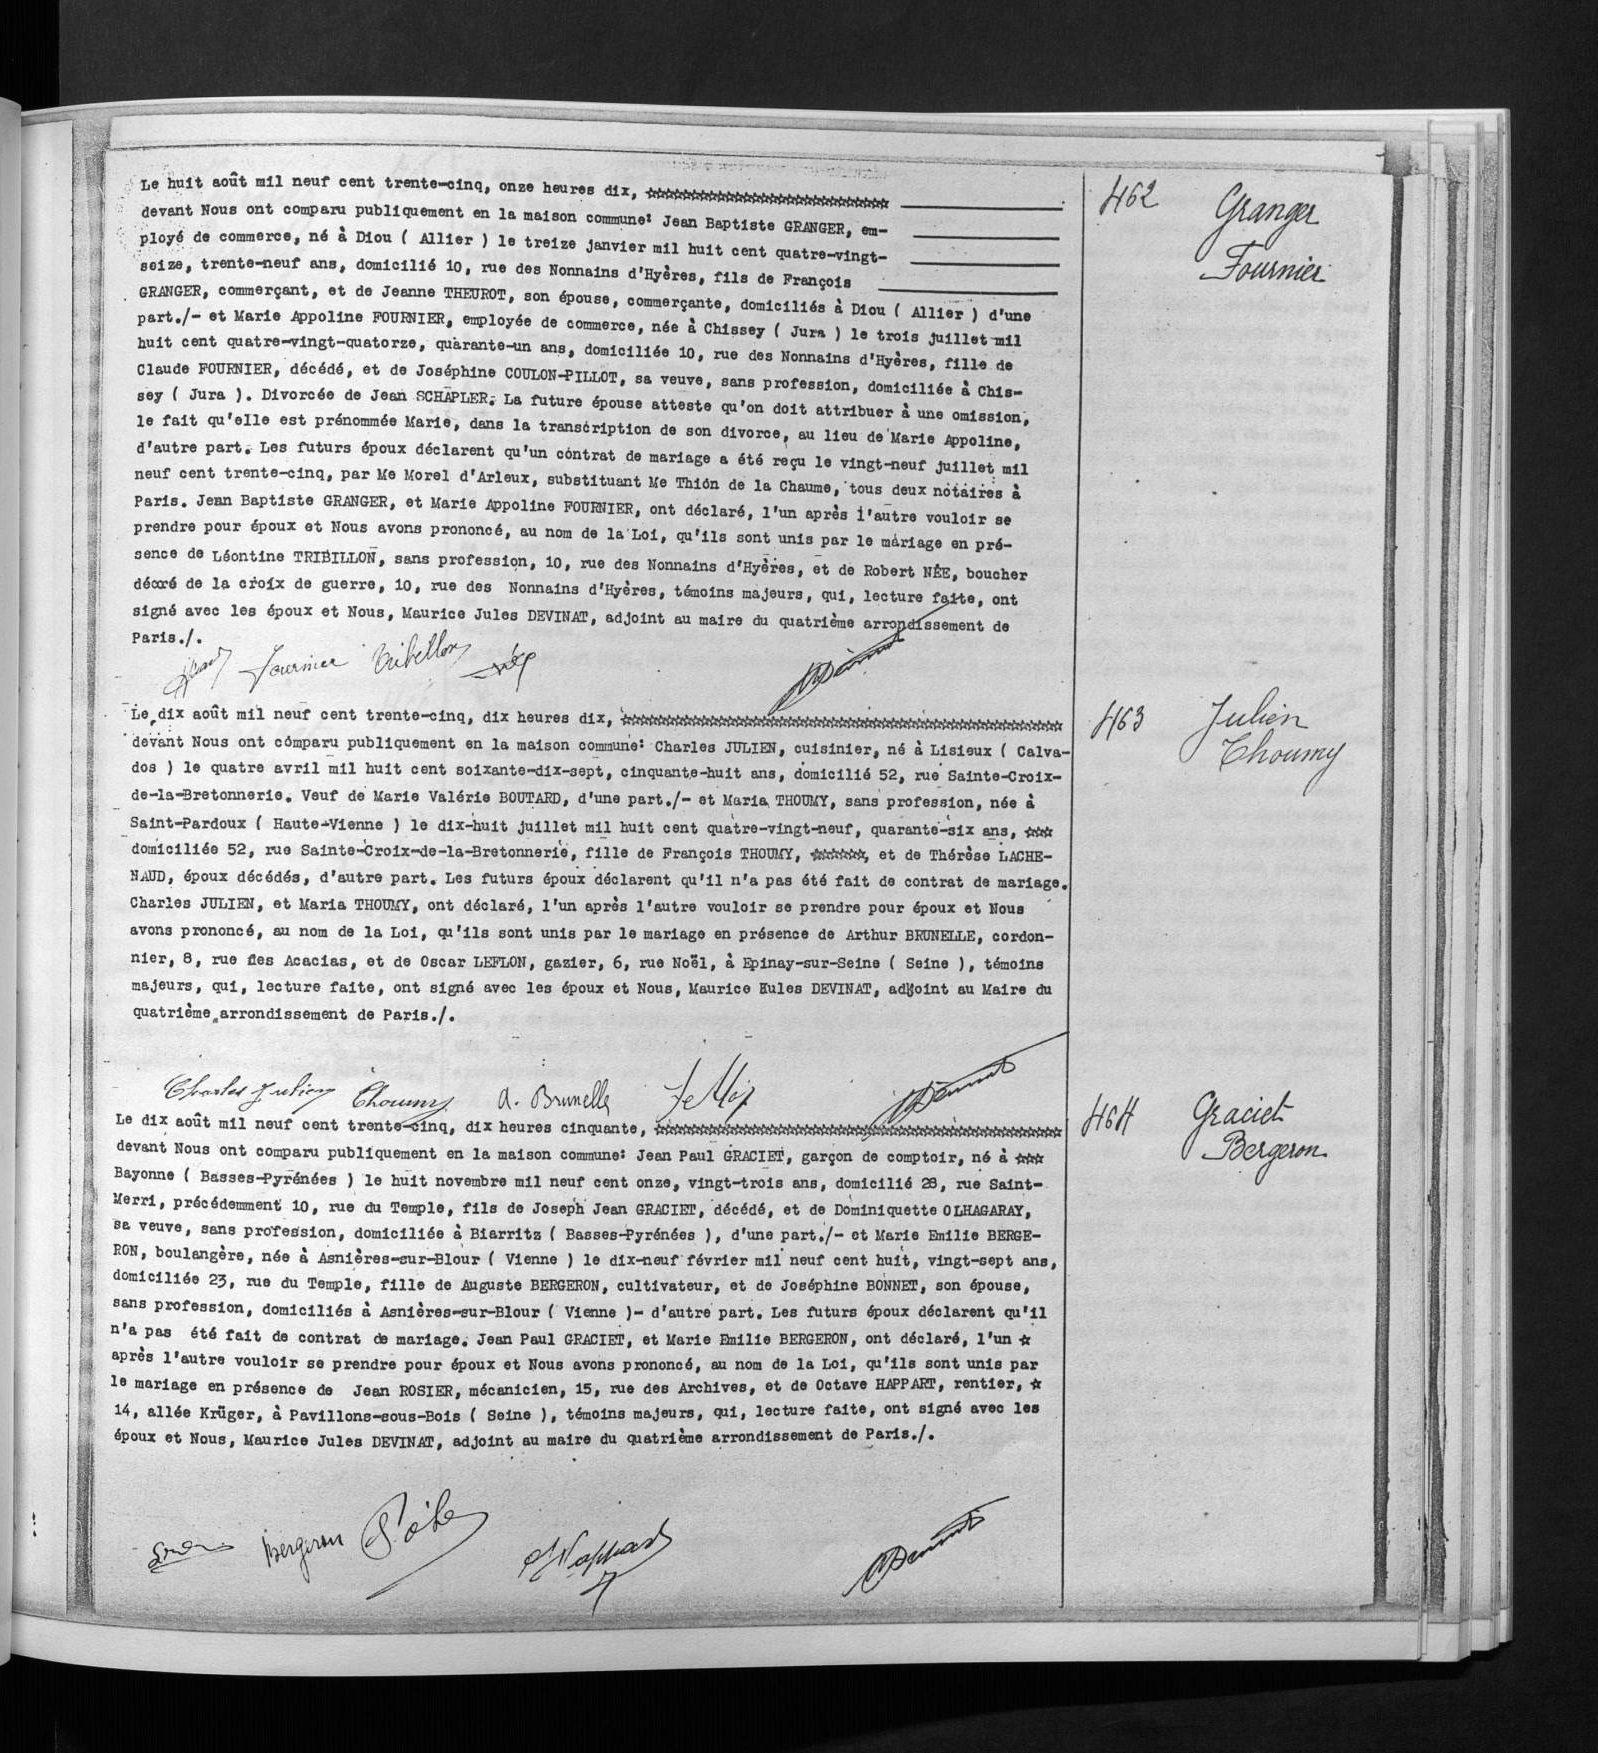Le huit août mil neuf cent trente-cinq, onze heures dix, ****-
devant Nous ont comparu publiquement en la maison commune: Jean Baptiste GRANGER, em -
mployé de commerce, né à Diou (Allier) le treize janvier mil huit cent quatre-vingt-
seize, trente-neuf ans, domicilié 10, rue des Nonnais d'Hyères, fils de François -
GRANGER, commerçant, et de Jeanne THEUROT, son épouse, commerçante, domiciliés à Diou (Allier) d'une
part ./-et Marie Appoline FOURNIER, employée de commerce, née à Chissey (Jura) le trois juillet mil
huit cent quatre-vingt-quatorze, quarante-un ans, domiciliée 10, rue des Nonnais d'Hyères, fille de
Claude FOURNIER, décédé, et de Joséphine COULON-PILLOT, sa veuve, sans profession, domiciliée à Chis-
sey (Jura). Divorcée de Jean SCHAPLER. La future épouse atteste qu'on doit attribuer à une amission,
le fait qu'elle est prénommée Marie, dans la transcription de son divorce, au lieu de Marie Appoline,
d'autre part. Les futurs époux déclarent qu'un contrat de mariage a été reçu le vingt-neuf juillet mil
neuf cent trente-cinq, par Me Morel d'Arleux, substituant Me Thion de la Chaume, tous deux notaires à
Paris. Jean Baptiste GRANGER, et Marie Appoline FOURNIER, ont déclaré, l'un après l'autre vouloir se
prendre pour époux et Nous avons prononcé, au nom de la Loi, qu'ils sont unis par le mariage en pré-
sence de Léontine TRIBILLON, sans profession, 10, rue des Nonnains d'Hyères, et de Robert NEE, boucher
décoré de la croix de guerre, 10, rue des Nonnains d'Hyères, témoins majeurs, qui, lecture faite, ont
signé avec les époux et Nous, Maurice Jules DEVINAT, adjoint au maire du quatrième arrondissement de
Paris ./.
Le dix août mil neuf cent trente-cinq, dix heures dix, ****
devant Nous ont comparu publiquement en la maison commune: Charles JULIEN, cuisinier, né à Lisieux (Calva-
dos) le quatre avril mil huit cent soixante-dix-sept, cinquante-huit ans, domicilié 52, rue Sainte-Croix-
de-la-Bretonnerie. Veuf de Marie Valérie BOUTARD, d'une part ./-et Maria THOUMY, sans profession, née à
Saint-Pardoux (Haute-Vienne) le dix-huit juillet mil huit cent quatre-vingt-neuf, quarante-six ans, ***
domiciliée 52, rue Sainte-Croix-de-la-Bretonnerie, fille de François THOUMY, ****, et de Thérèse LACHE-
NAUD, époux décédés, d'autre part. Les futurs époux déclarent qu'il n'a pas été fait de contrat de mariage.
Charles JULIEN, et Maria THOUMY, ont déclaré, l'un après l'autre vouloir se prendre pour époux et Nous
avons prononcé, au nom de la Loi, qu'il sont unis par le mariage en présence de Arthur BRUNELLE, cordon-
nier, 8, rue des Acacias, et de Oscar LEFLON, gazier, 6, rue Noël, à Epinay-sur-Seine (Seine), témoins
majeurs, qui, lecture faite, ont signé avec les époux et Nous, Maurice Hules DEVINAT, adjoint au Maire du
quatrième arrondissement de Paris ./.
Le dix août mil neuf cent trente-cinq, dix heures cinquante, ****
devant Nous ont comparu publiquement en la maison commune: Jean Paul GRACIET, garçon de comptoir, né à **
Bayonne (Basses-Pyrénées) le huit novembre mil neuf cent onze, vingt-trois ans, domicilié 28, rue Saint-
Merri, précédemment 10, rue du Temple, fils de Joseph Jean GRACIET, décédé, et de Dominiquette OLHARGARAY,
sa veuve, sans profession, domiciliée à Biarritz (Basses-Pyrénées), d'une part ./-et Marie Emilie BERGE-
RON, boulangère, née à Asnière-sur-Blour (Vienne) le dix-neuf février mil neuf cent huit, vingt-sept ans,
domiciliée 23, rue du Temple, fille de Auguste BERGERON, cultivateur, et de Joséphine BONNET, son épouse,
sans profession, domiciliés à Asnière-sur-Blour (Vienne)-d'autre part. Les futurs époux déclarent qu'il
n'a pas été fait de contrat de mariage. Jean Paul GRACIET, et Marie Emilie BERGERON, ont déclaré, l'un *
après l'autre vouloir se prendre pour époux et Nous avons prononcé, au nom de la Loi, qu'ils sont unis par
le mariage en présence de Jean ROSIER, mécanicien, 15, rue des Archives, et de Octave HAPPART, rentier, *
14, allée Krüger, à Pavillons-sous-Bois (Seine), témoins majeurs, qui, lecture faite, ont signé avec les
époux et Nous, Maurice Jules DEVINAT, adjoint au maire du quatrième arrondissement de Paris ./.
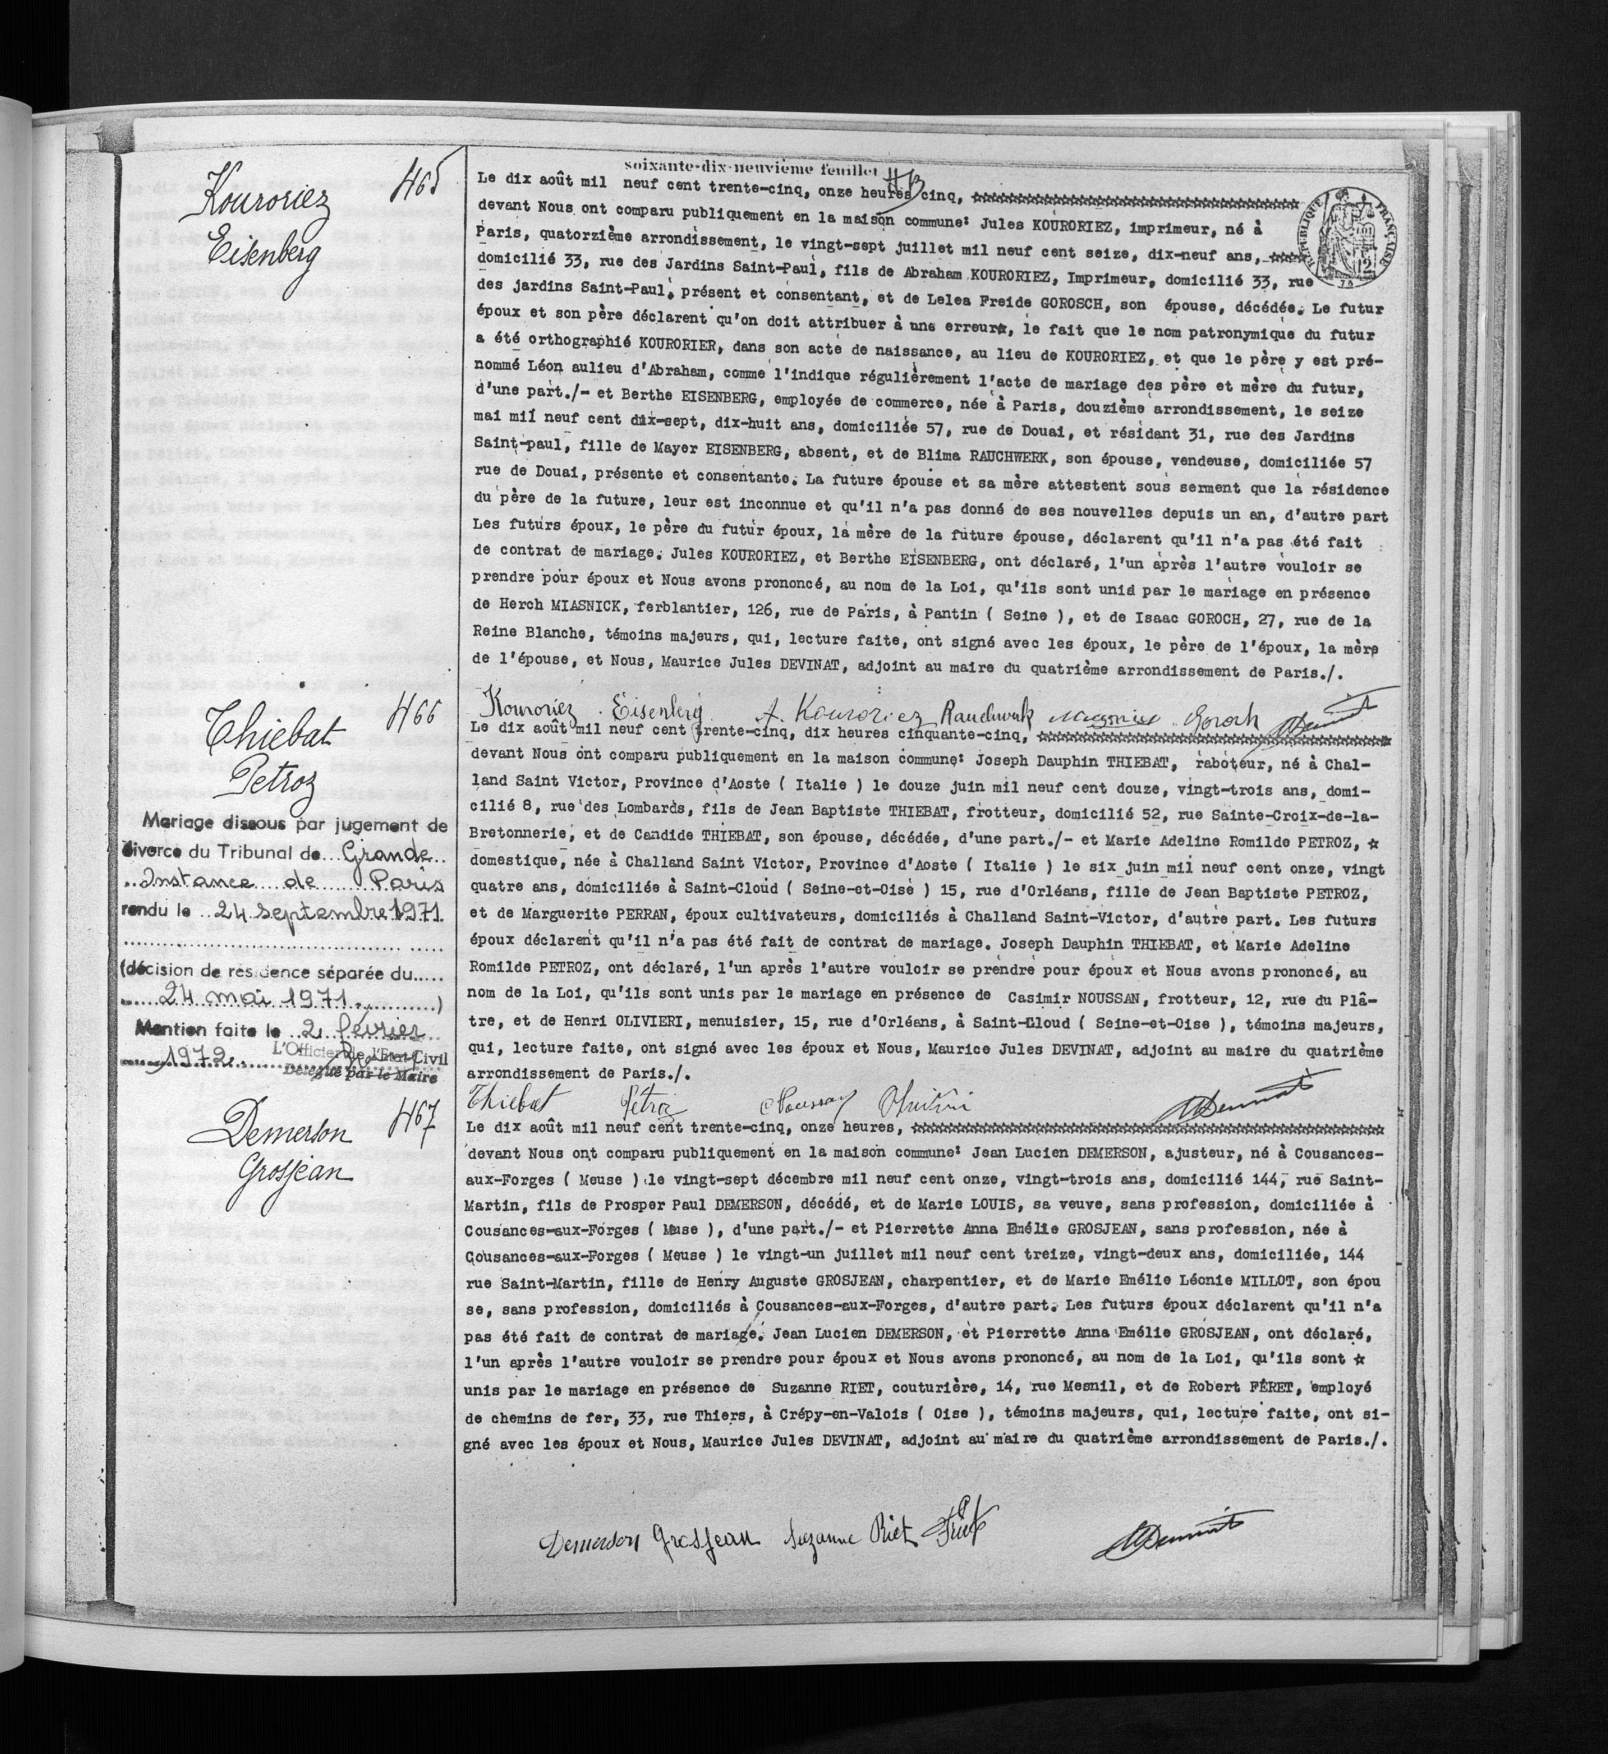Le dix août mil neuf cent trente-cinq, onze heures cinq, ****
devant Nous ont comparu publiquement en la maison commune: Jules KOURORIEZ, imprimeur, né à
Paris, quatorzième arrondissement, le vingt-sept juillet mil neuf cent seize, dix-neuf ans, ***
domicilié 33, rue des Jardins Saint-Paul, fils de Abraham KOURORIEZ, Imprimeur, domicilié 33, rue
des jardins Saint-Paul, présent et consentant, et de Lelea Freide GORSCH, son épouse, décédés. Le futur
époux et son père déclarent qu'on doit attribuer à une erreur *, le fait que le nom patronymique du futur
a été orthographie KOURORIER, sans son acte de naissance, au lieu de KOURORIEZ, et que le père y est pré-
nommé Léon aulieu d'Abraham, comme l'indique régulièrement l'acte de mariage des père et mère du futur,
d'une part ./-et Berthe EISENBERG, employée de commerce, née à Paris, douzième arrondissement, le seize
mai mil neuf cent dix-sept, dix-huit ans, domiciliée 57, rue de Douai, et résidant 31, rue des Jardins
Saint-paul, fille de Mayer EISENBERG, absent, et de Bloma RAUCHWERK, son épouse, vendeuse, domiciliée 57
rue de Douai, présente et consentante. La future épouse et sa mère attestent sous serment que la résidence
du père de la future, leur est inconnué et qu'il n'a pas donné de ses nouvelles depuis un an, d'autre part
Les futurs époux, le père du futur époux, la mère de la future épouse, déclarent qu'il n'a pas été fait
de contrat de mairage. Jules KOURORIEZ, et Berthe EISENBERG, ont déclaré l'un après l'autre vouloir se
prendre pour époux et Nous avons pronnoncé, au nom de la Loi, qu'ils sont unis par le mariage en présence
de Herch MIASNICK, ferblantier, 136, rue de Paris, à Pantin (Seine), et de Isaac GOROCH, 27, rue de la
Reine Blanche, témoins majeurs, qui, lecture faite, ont signé avec les époux, le père de l'époux, la mère
de l'épouse, et Nous, Maurice Jules DEVINAT, adjoint au maire du quatrième arrondissement de Paris ./.
Le dix août mil neuf cent trente-cinq, dix heures cinquante-cinq, ****
devant Nous ont comparu publiquement en la maison commune: Joseph Dauphin THIEBAT, reboteur, né à Chal-
land Saint Victore, Province d'Aoste (Italie) le douze juin mil neuf cent douze, vingt-trois ans, domi-
cilié 8, rue des Lombards, fils de Jean Baptiste THIEBAT, frotteur, domicilié 51, rue Sainte-Croix-de-la-
Bretonnerie, et de Candide THYEBAT, son épouse, décédée, d'une part ./-Et Marie Adeline Romilde PETROZ ,*
domestique, née à Challand Saint Victor, Province d'Aoste (Italie à le six juin mil neuf cent onze, vingt
quatre ans, domiciliée à Saint-Cloud (Seine-et-Oise) 15, rue d'Orléans, fille de Jean Baptiste PETROZ,
et de Marguerite PERRAN, époux cultivateurs, domiciliés à Challand Saint-Victor, d'autre part. Les futurs
époux déclarent qu'il n'a pas été fait de contrat de mariage. Joseph Dauphin THIEBAT, et Marie Adeline
Romilde PETROZ, ont déclaré, l'un après l'autre voulior se prendre pour époux et Nous avons prononcé, au
nom de la Loi, qu'ils sont unis par le mariage en présence de Casimir NOUSSAN, frotteur, 12, rue du Plâ-
tre, et de Henri OLIVIERI, menuisier, 15, rue d'Orléans, à Saint-Cloud (Seine-et-Oise), témois majeurs,
qui, lecture faite, ont signé avec les époux et Nous, Maurice Jules DEVINAT, adjoint au maire du quatrième
arrondissement de Paris ./.
Le dix août mil neuf cent trente-cinq, onze heures ,****
devant Nous ont comparu publiquement en la maison commune: Jean Lucien DEMERSON, ajusteur, né à Cousances-
aux-Forges (Meuse) le vingt-sept décembre mil neuf cent onze, vingt-trois ans, domicilié 144, rue Saint-
Martin, fils de Prosper Paul DEMERSON, décédé, et de Marie LOUIS, sa veuve, sans profession, domiciliée à
Cousances-aux-Forges (Meuse), d'une part ./-et Pierrette Anna Emélie GROSJEAN, sans profession, née à
Cousances-aux-Forges (Meuse) le vingt-un juillet mil neuf cent treize, vingt-deux ans, domiciliée, 144
rue Saint-Martin, fille de Henry Auguste GROSJEAN, charpentier, et de Marie Emélie Léonie MILLOT, son épou
se, sans profession, domiciliés à Cousances-aux-Forges, d'autre part. Les futurs époux déclarent qu'il n'a
pas été fait de contrat de mariage. Jean Lucien DEMERSON, et Pierrette Anna Emélie GROSJEAN, ont déclaré,
l'un après l'autre vouloir se prendre pour époux et Nous avons prononcé, au nom de la Loi, qu'ils sont *
unis par le mariage en présence de Suzanne RIET, couturière, 14, rue Masnil, et de Robert FERET, employé
de chemins de fer, 33, rue Thiers, à Crépy-en-Valois (Oise), témoins majeurs, qui, lecture faite, ont si-
gné avec les époux et Nous, Maurice Jules DEVINAT, adjoint au maire du quatrième arrondissement de Paris ./.